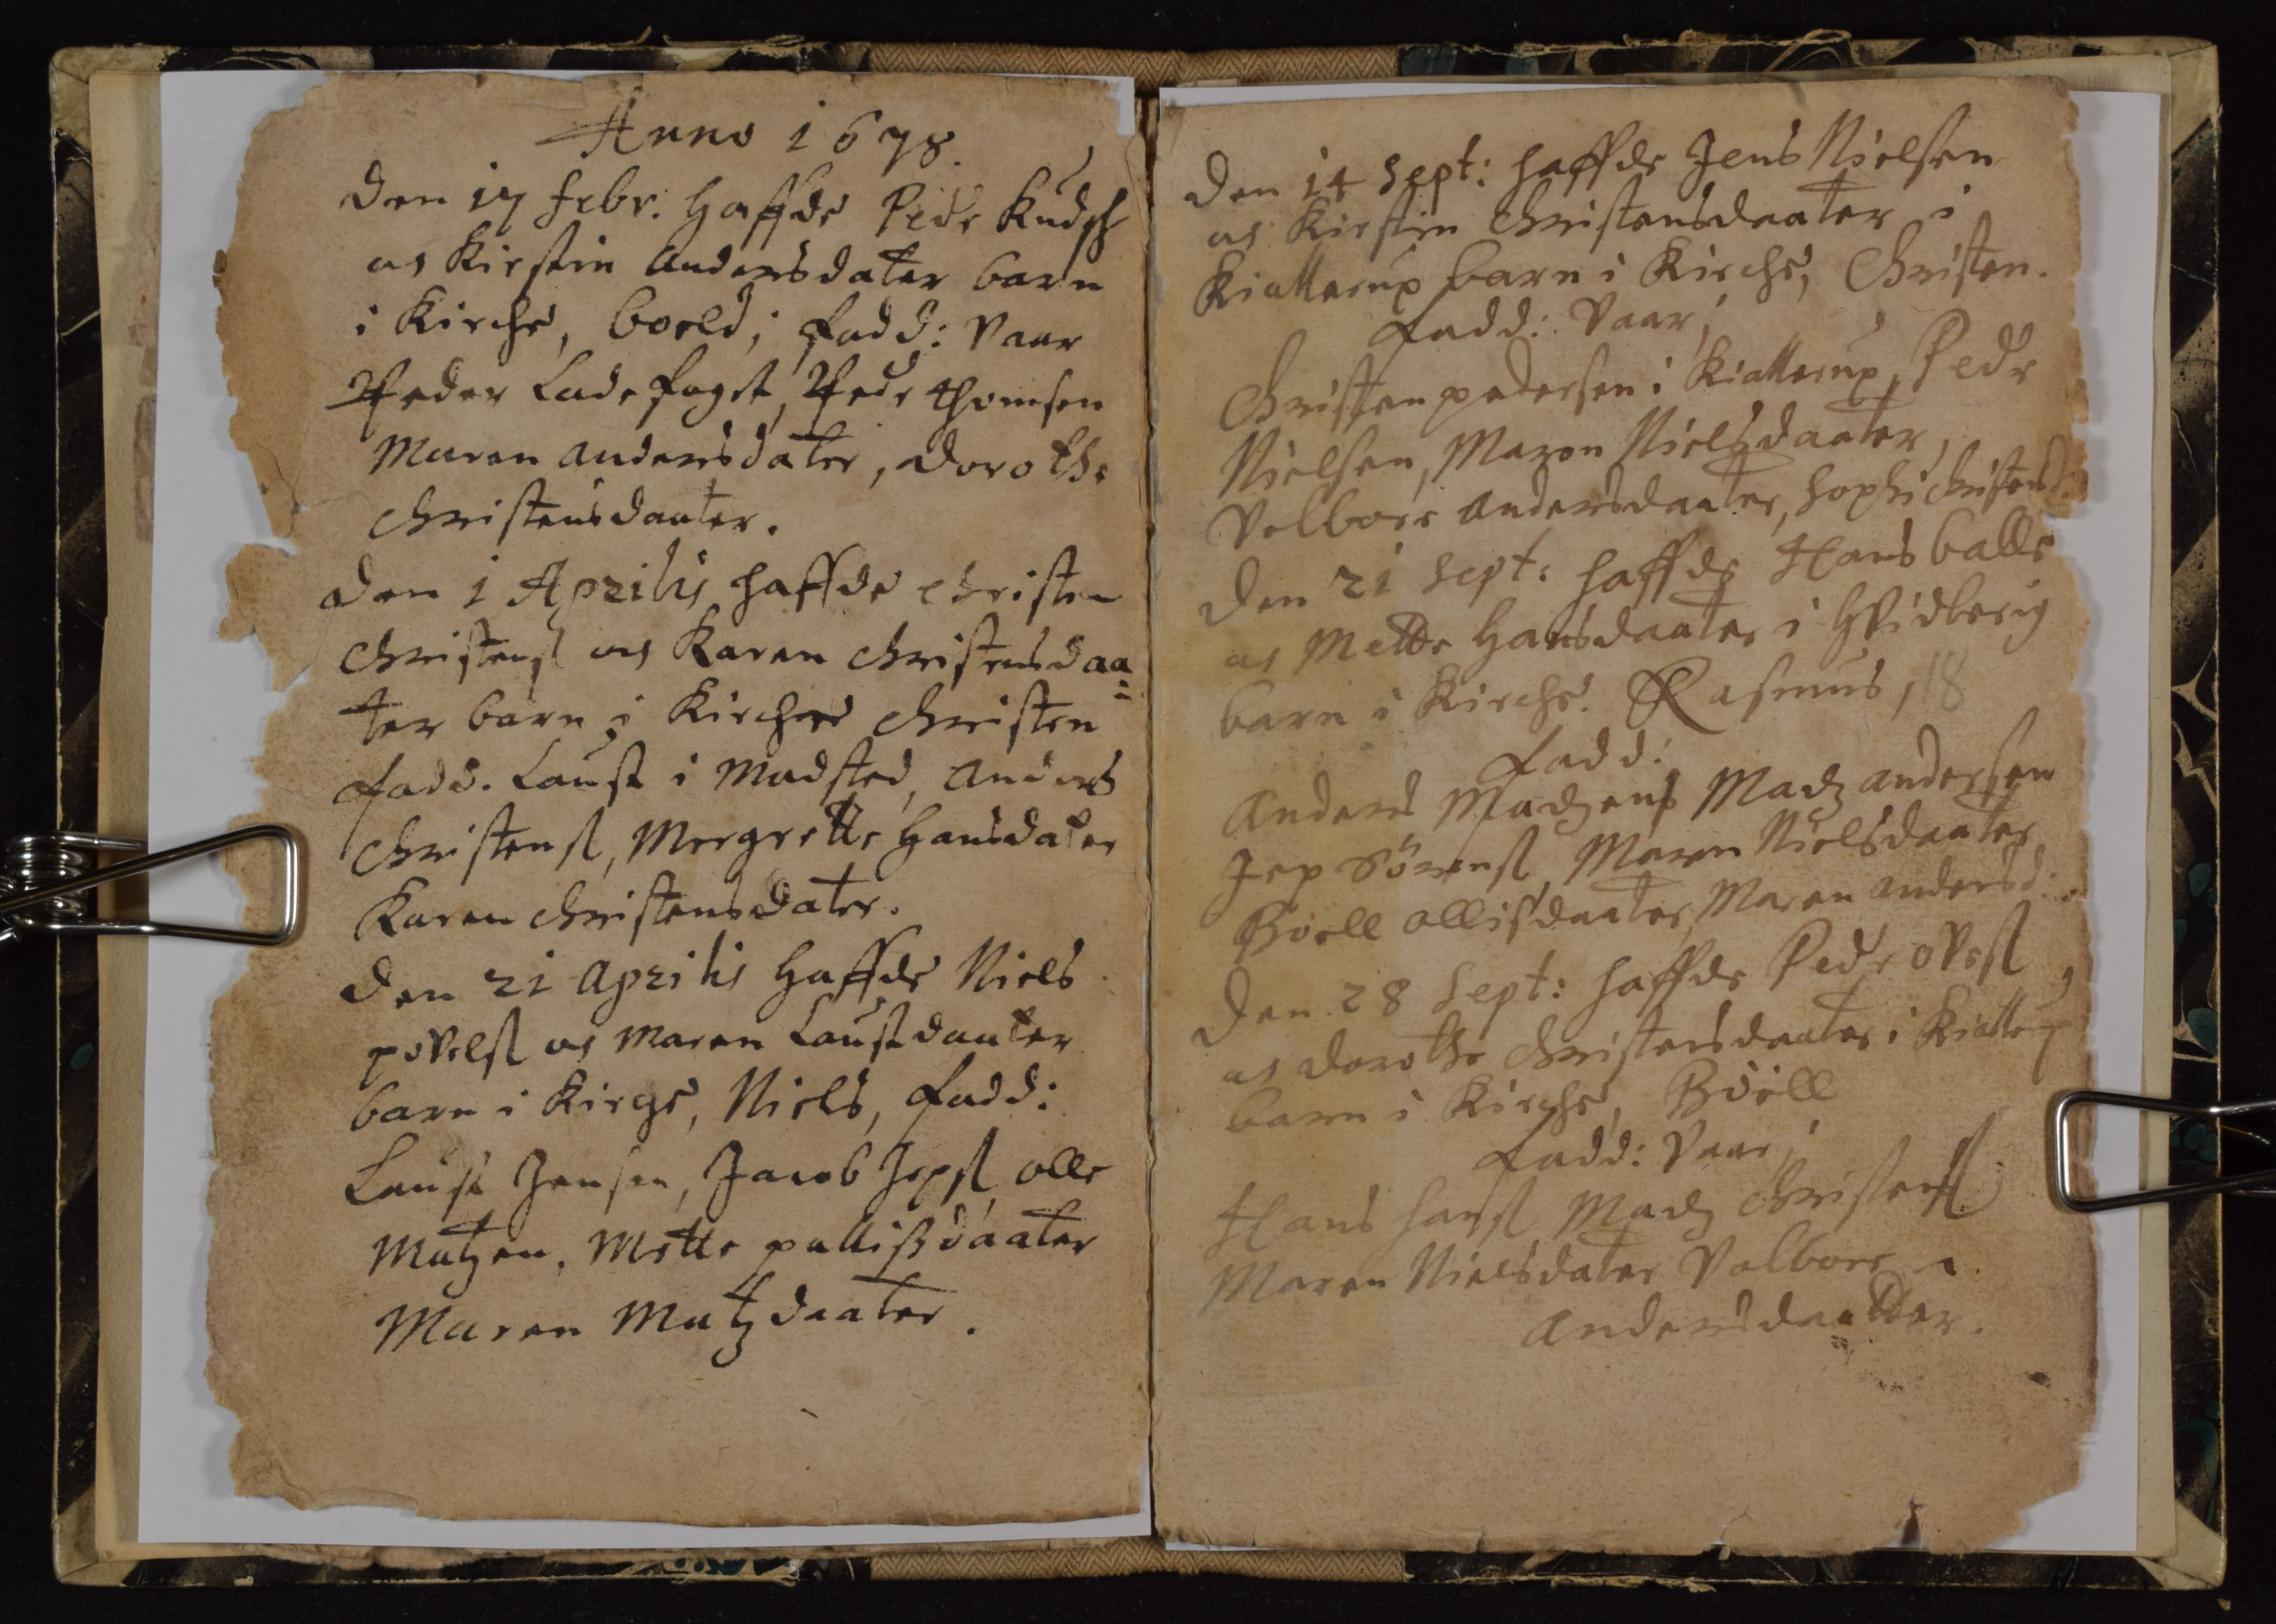Anno 1678.
Den 17 Febr: Haffde Pedr Kudsh
och Kirstin Andersdater barn
i Kirche boeld; Fadd: vaar
Peder Ladefoget Pedr thomsen
Maren Andersdater, Dorothe
christensdauter.
Den 1 Aprilis haffde Christen
Christens och Karen Christensdaa¬
ter barn i Kirche Christen
Fadd. Laust i Madsted, Anders
Christens, Mergrette Hansdater
Karen Christensdater.
Den 21 Aprilis Haffde Niels
povels och Maren Laustdauter
barn i Kirche, Niels, Fadd:
Laust Jensen, Jacob Jeps, Olle
Matzen, Mette pallissdaater
Maren Matzdaater
Den 14 Sept: haffde Jens Nielsen
och Kirstin christensdaater i
Kiallarup barn i Kirche Christen.
Fadd: vaar
Christen pedersen i Kiallerup Pedr
Nielsen, Maren Nielsdaater
Velbore Andersdaater, Sophe Christensd:
Den 21 Sept: haffde Hans balle
och Mette Hansdaater i Hvidberig
barn i Kirche. Rasmus,
Fadd:
Anders Madzens Madz Andersen
Jep Sørens, Maren Nielsdaater
Boell ollisdatter, Maren Andersd:
Den 28 Sept: haffde Pedr Oves
och Dorothe christensdaater i Kiallerup
barn i Kirche, Boell
Fadd: vaar
Hans hans Madz christens
Maren Nielsdater Valbore
Andersdaatter.
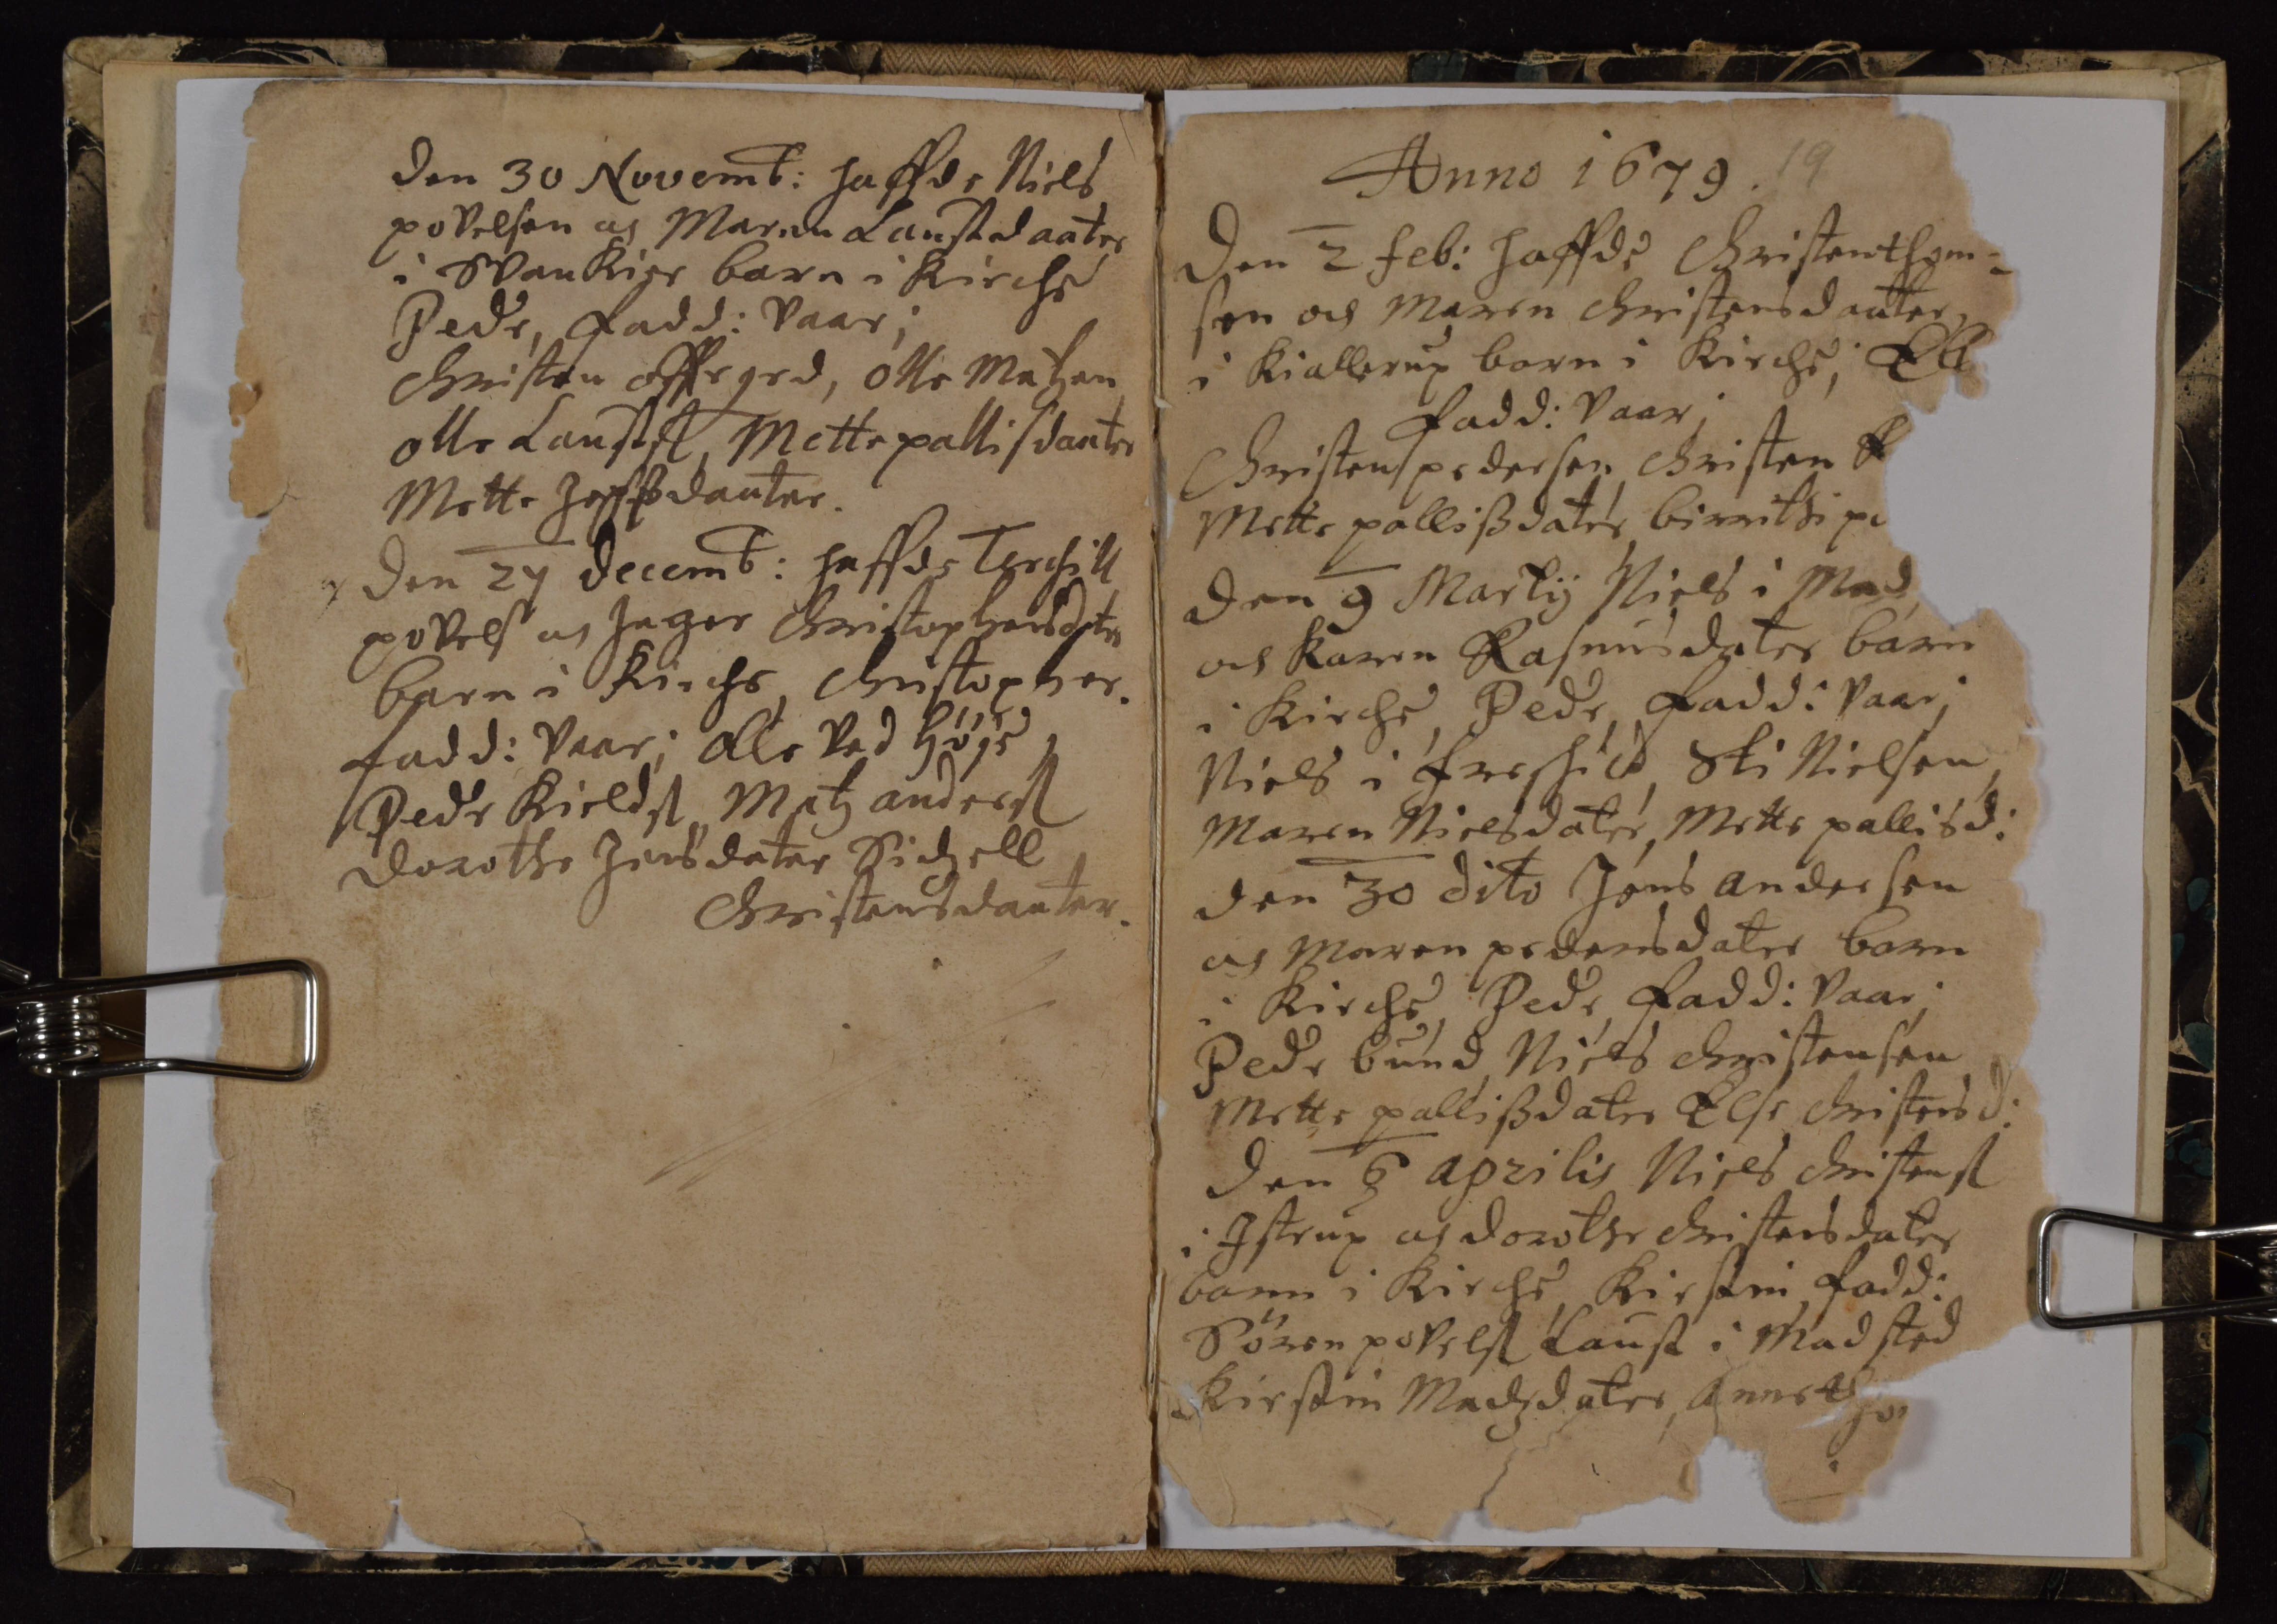Den 30 Novemb: haffde Niels
povelsen och Maren Laustdaater
i Svankier barn i Kirche
Pede, Fadd: vaar;
Christen offrgrd. olle Matzen
Olle Lausts, Mette pallisdauter
Mette Jepssdauter.
Den 27 Decemb: haffdes Terchill
povels och Inger christophersdater
barn Kirche christopher
Fadd: vaar, oplle ved Højs
Pedr Kields Matz anders
Dorothe Jensdater Sidzell
christensdauter
Anno 1679
Den 2 Feb: haffde Christen thom¬
sen och Maren Christensdauter
i Kiallerup barn i Kirche, El  
Fadd: vaar
Christens pedersen Christen F
Mette pallissdater Birrithe p  
den 9 Martij Niels i Mad
och Karen Rasmusdater barn
i Kirche, Pedr, Fadd: vaar
Niels i Fresheld, Sti Nielsen
Maren Nielsdater Mette pallisd:
en 30 dito Jens Andersen
och Maren pedersdater barn
i Kirche, Pedr, Fadd: vaar;
Pedr bund Niels christensen
Mette pallissdater Else Christensd:
Den 6 Aprilis Niels Christens
i Istrup och Dorothe Christensdater
barn i Kirche Kirstin Fadd:
Søren povels Laust i Madsted
Kirstin Madzdater Anne th